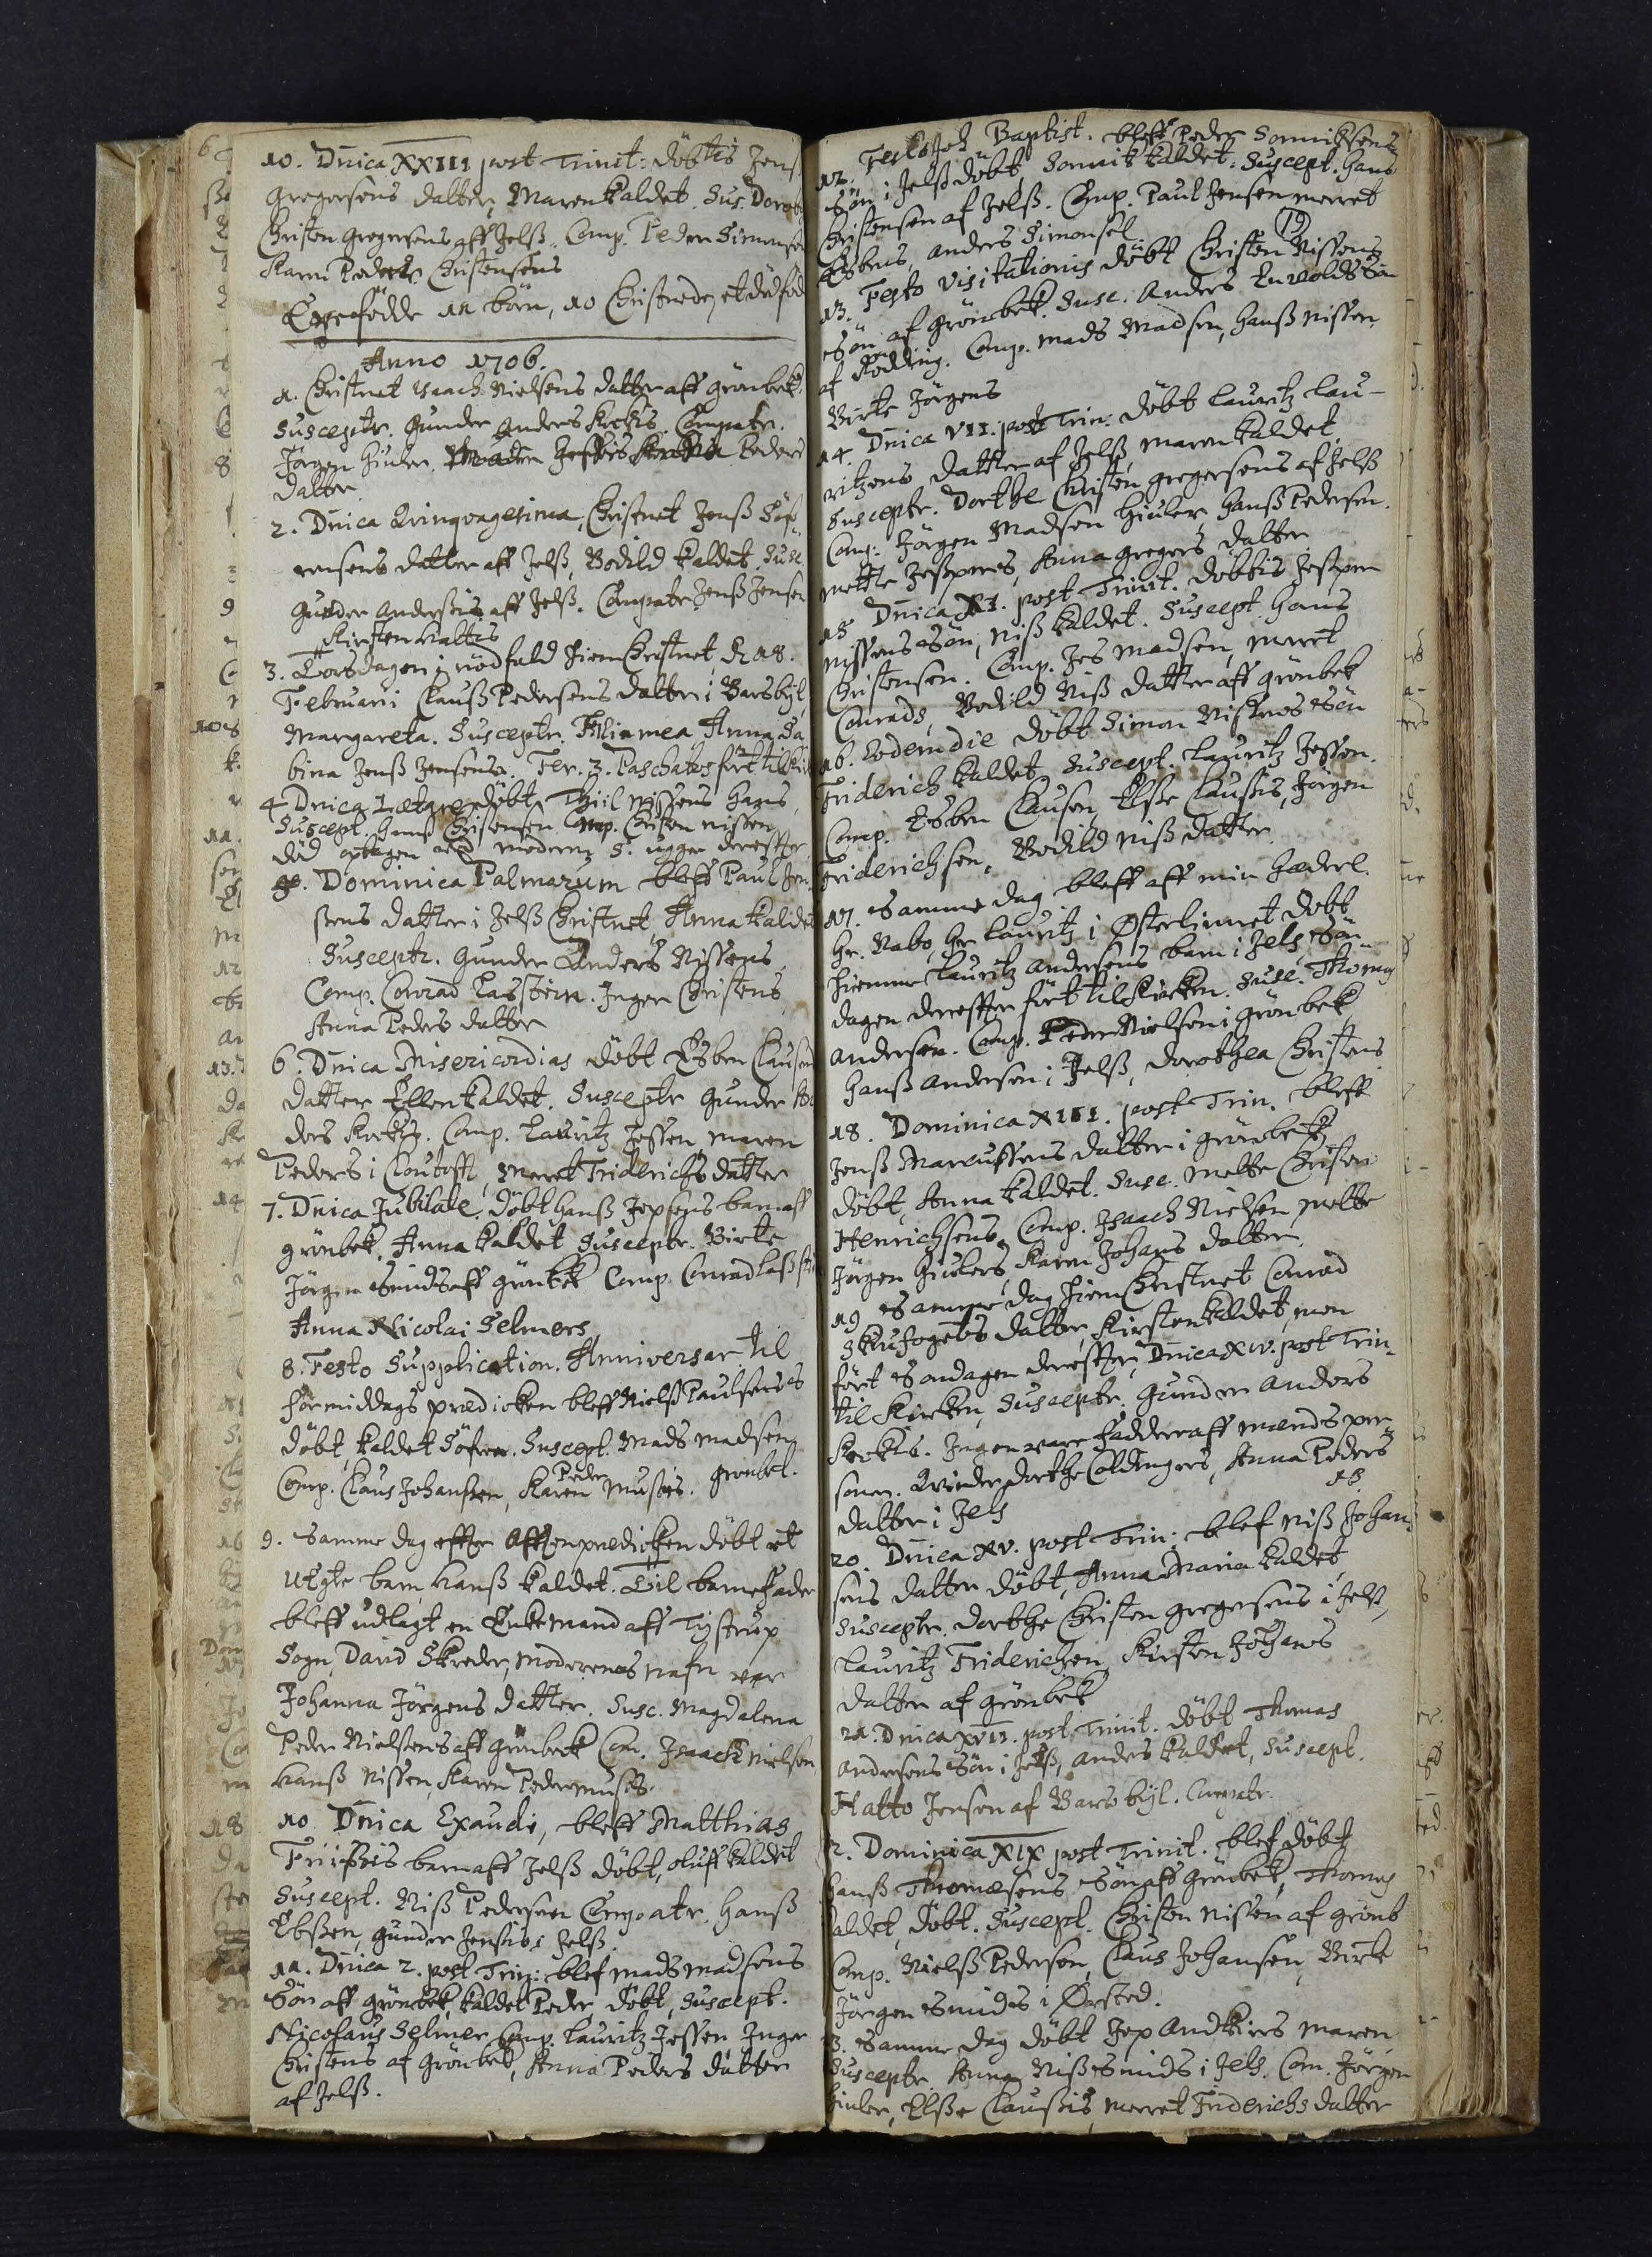10. Dnica XXIII post Trinit: døbtis Jens
Gregersens datter, Maren kaldet. Sus. Dorot 
Christen Gregersens aff Jelss. Comp. Peder Simonsen
Karen Peder Christensens.
Ere## fødde 11 børn, 10 Christnede, et dødfød
Anno 1706.
1. Christnet Isaach Nielsens datter aff Grønbek.
Susceptr. Gunder Anders Kockis. Compatr.
Jørgen Hiuler. Maren Jessis. Kirsten## Peders
datter
2. Dnica Qvinqvagesima, Christnet Jens Søf¬
rensens datter aff Jelss, Bodild kaldet. Susc.
Gunder Andersis aff Jelss. Compatr. Jens Jensen
Kirsten Hattis
3. Torsdagen i nødfald hiem Christnet d 18.
Februari Clauss Pedersens datter i Barsbyl
Margereta. Susceptr. Filia mea Anna Sa
bina Jens Jensens. Fer. 3. Paschatos ført til kir
4. Dnica Lætare døbt Thiil Nissens Hans
Suscept. Hans Christensen. Comp. Christen Nissen
Død optagen af moderen 5. ugger dereffter
5. Dominica Palmarum bleff Paul Jen¬
ssens datter i Jelss Christnet, Anna kaldet
Susceptr. Gunder Anders Nissens
Comp. Conrad Lasstein. Inger Christens
Anna Peders Datter
6. Dnica Misericordias døbt Esben Clausens
datter Ellen kaldet. Susceptr. Gunder An
ders Kockis. Comp. Lauritz Jessen, Maren
Peders i Cloutofft, Merret Friderichs datter
7. Dnica Jubilate. døbt Hanss Jepsens barn aff
Grønbek. Anna Kaldet. Susceptr. Birte
Jørgen Smids aff Grønbek Comp. Conrad Lasste
Anna Nicolai Selmers
8. Festo Supplication. Anniversar til
formiddags prædiken bleff Niels Paulsens
døbt, kaldet Søfren. Suscept. Mads Madsen
Peder
Comp. Claus Johansen, Karen Nissis. Grønbek.
9. Samme dag effter aftenprædiken døbt et
uEgte barn Hanss kaldet. Til barnefader
bleff udlagt en Enkemand aff Tystrup
Sogn David Skreder, Moderens nafn var
Johanna Jørgens datter. Susc. Magdalena
Peder Nielsens aff Grønbek Com. Isaach Nielsen,
Hans Nissen, Karen
10. Dnica Exaudi , bleff Matthias
Friissis barn aff Jelss døbt, Oluff kaldet
Suscept. Niss Pedersen Compatr. Hanss
Ebssen, Gunder Jensis i Jelss
11. Dnica 2. post Trin. blef Mads Madsens
Søn aff Grønbek, kaldet Peder døbt, Suscept.
Nicolaus Selmer. Comp. Lauritz Jessen, Inger
Christens af Grønbek, Anna Peders datter
af Jelss.
19
12. Festo Joh Baptist. bleff Peder Sonniksens
Søn i Jelss døbt, Sonnik kaldet. Suscept. Hans
Christensen af Jelss. Comp. Paul Jensen Merret
Esbens, Anders Simonsel
13. Festo Visitationis døbt Christen Nissens
Søn af Grønbek. Susc. Anders Envolds Søn
af Rødding. Comp. Mads Madsen, Hans Nissen,
Birte Jørgens
14. Dnica VII. post Trin. døbt Lauritz Lau¬
ritzens datter af Jelss, Maren kaldet
Susceptr. Dorthe Christen Gregersens af Jelss
Comp: Jørgen Madsen Hiuler, Hanss Pedersen.
Mette Jesspers, Anna Gregers datter
15 Dnica XI. post Trinit. døbtis Jesper
Nissens Søn, Niss kaldet. Suscept. Hans
Christensen. Comp. Jes Madsen, Merret
Conrads, Bodild Niss datter aff Grønbek
16. Eodem die døbt Simon Nissens Søn
Friderich kaldet. Suscept. Lauritz Jessen.
Comp. Esben Clausen, Elsse Claussis, Jørgen
Friderichsen. Bodild Niss datter
17. Samme dag bleff aff min Hæderl.
Hr. Nabo, Hr Lauritz i Østerlinnet døbt
hiemme Lauritz Andersens barn i Jels Søn
dagen dereffter ført til Kirken. Susc. Thomas
Andersen. Comp. Peder Nielsen i Grønbek
Hans Andersen i Jelss, Dorothea Christens.
18. Dominica XIII. post Trin. bleff
Jenss Marcussens datter i Grønbek
døbt, Anna kaldet. Susc. Mette Christen
Henrichsens. Comp. Isaach Nielsen, Mette
Jørgen Hiulers, Karen Johans datter
19. Samme dag hiem Christnet Conrad
Skoufogets datter, Kirsten kaldet, men
ført Søndagen dereffter, Dnica XIV. post Trin
til Kirken, susceptr. Gunder Anders
Kockis. Ingen vare fadder aff mands per¬
soner. Qvinde Dorthe Coldingers, Anna Peders
datter i Jels
##
20. Dnica XV. post Trin. blef Niss Johan
sens datter døbt, Anna Maria kaldet
Susceptr. Dorthe Christen Gregersens i Jelss
Lauritz Friderichsen, Kirsten Johans
datter af Grønbek
21. Dnica XVII. post Trinit. døbt Thomas
Andersens Søn i Jelss, Anders kaldet, suscept.
Hatto Jensen af Barsbyl. Compatr.
2. Dominica XIX post Trinit. blef døbt
Hans Thomæsens Søn aff Grønbek, Thomas
kaldet, døbt. Suscept. Christen Nissen af Grønb
Comp. Nielss Pedersen, Claus Johansen, Birte
Jørgen Smids i Ørsted.
3. Samme dag døbt Jep Andkiers Maren
Susceptr. Anna Niss Smids i Jels. Com. Jørgen
Hiuler, Elsse Claussis, Merret Friderichs datter